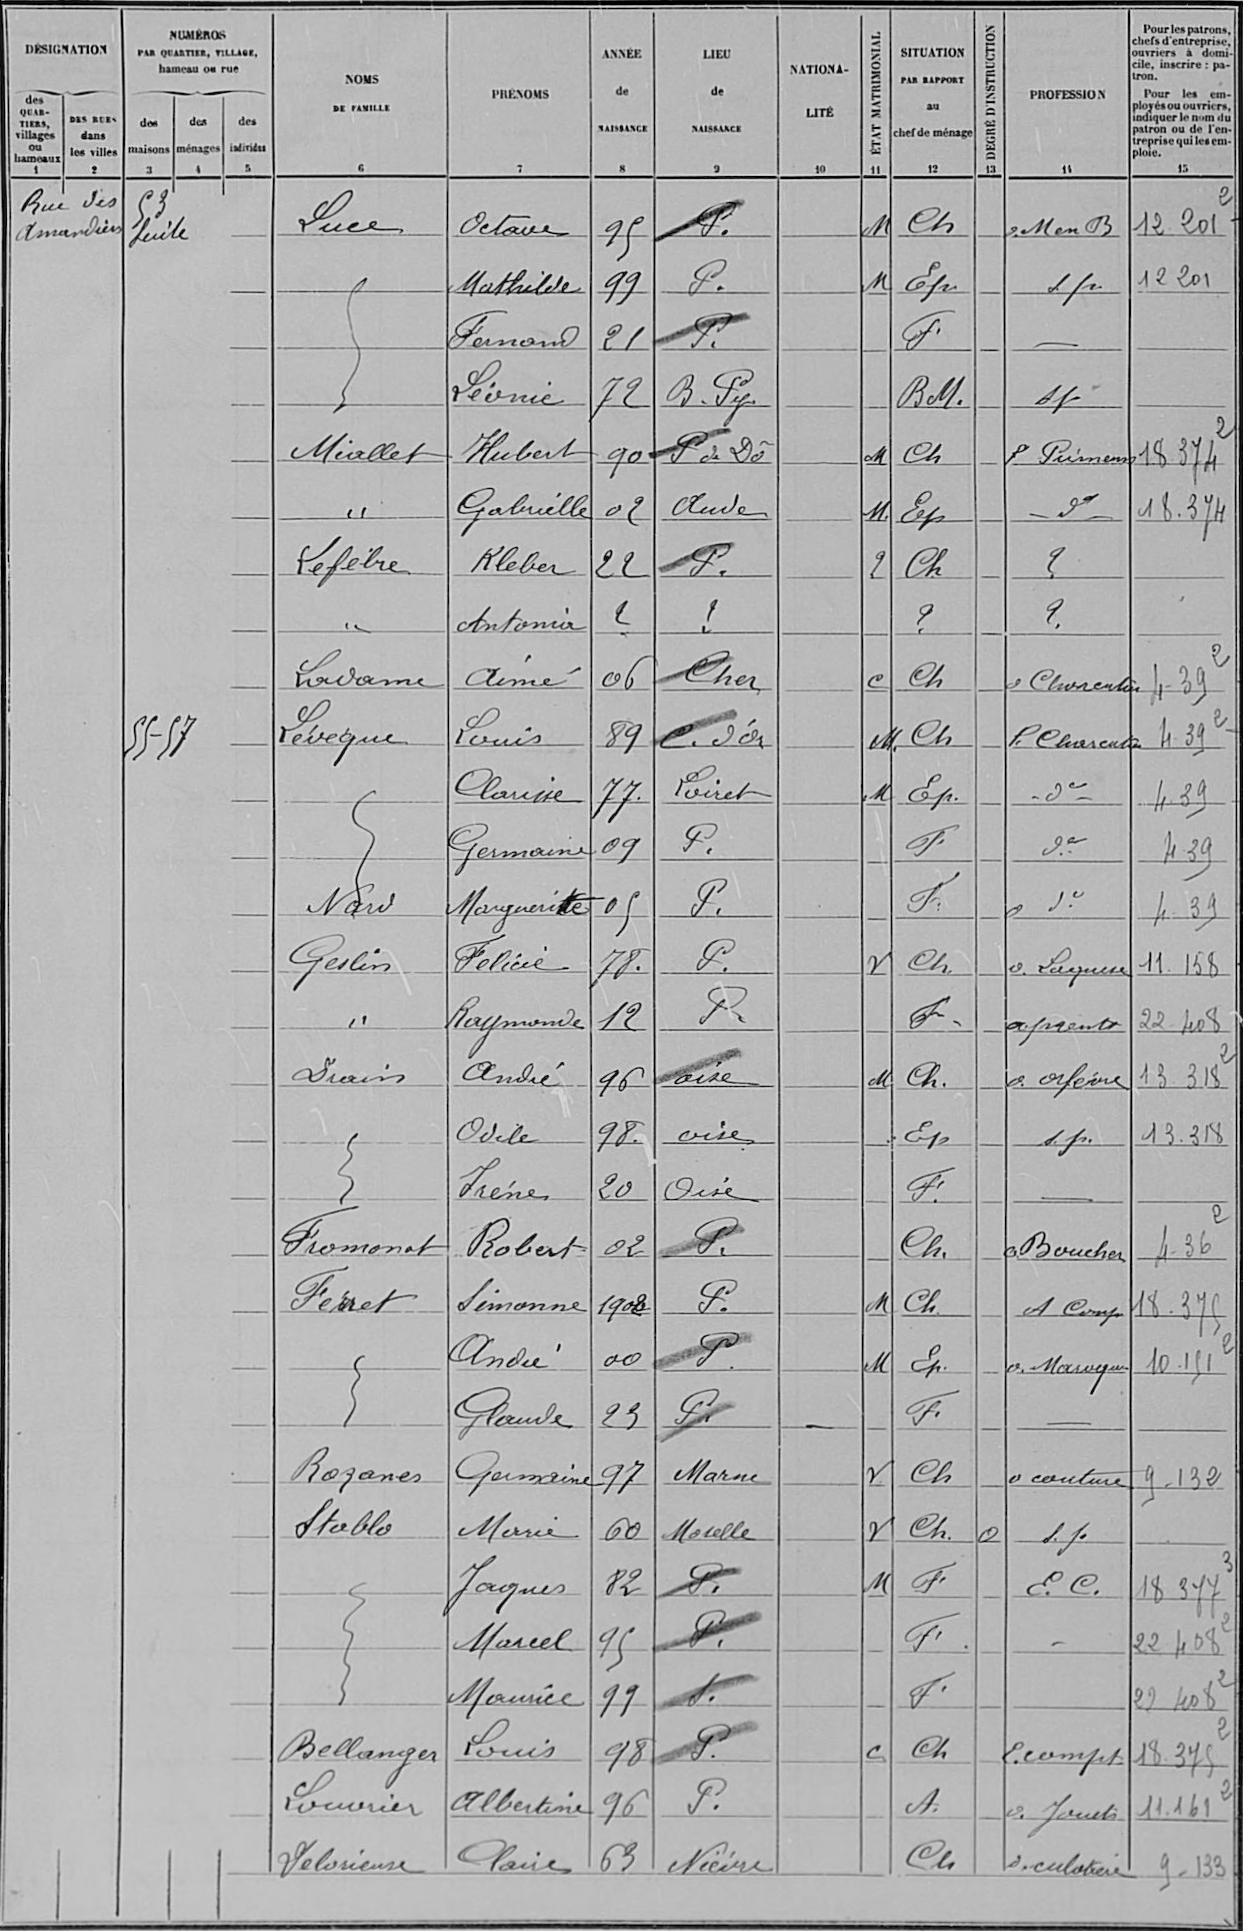Luce/Octave/95/P /¤/M/Ch/¤/Men B/12201
¤/Mathilde/99/P /¤/M/Ep/¤/s p/12201
¤/Fernand/21/P /¤/¤/F/¤/¤/¤
¤/Léonie/72/B Py /¤/¤/BM /¤/sp/¤
Miallet/Hubert/90/P de Do/¤/M/Ch/¤/P Primeurs/18374
''/Gabrielle/02/Aude/¤/M/Ep /¤/d°/18374
Lefebre/Kleber/22/P /¤/¤/Ch/¤/¤/¤
''/Antonin/¤/¤/¤/¤/¤/¤/¤/¤
Louvame/Aimé/06/Cher/¤/C/Ch/¤/o Charcutier/4 39
Léveque/Louis/89/C D'or/¤/M/Ch/¤/P Charcutier/4 39
¤/Clarisse/77/Loiret/¤/M/Ep/¤/d°/4 39
¤/Germaine/09/P /¤/¤/F/¤/d°/439
Naril/Marguerite/05/P /¤/¤/F/¤/d°/4 39
Geslin/Felicie/78/P /¤/V/Ch/¤/o Laqueuse/11158
''/Raymonde/12/P /¤/¤/F/¤/aprenti/22408
Drain/André/96/oise/¤/M/Ch /¤/o orfevre/13318
¤/Odile/98/oise/¤/¤/Ep/¤/s p /13318
¤/Irène/20/Oise/¤/¤/F/¤/¤/¤
Fromonat/Robert/02/P /¤/¤/Ch/¤/o Boucher/436
Fenet/Simonne/1908/P /¤/M/Ch/¤/A Comp/18375
¤/André/00/P /¤/M/Ep/¤/o Maroquin/10151
¤/Claude/23/P /¤/¤/F/¤/¤/¤
Roganes/Germaine/97/Marne/¤/V/Ch/¤/o couture/9132
Stablo/Marie/60/Moselle/¤/V/Ch/O/s p /¤
¤/Jacques/82/P /¤/M/F /¤/E C /18377
¤/Marcel/95/P /¤/¤/F/¤/¤/22408
¤/Maurice/99/P /¤/¤/F/¤/¤/22408
Bellanger/Louis/98/P /¤/c/Ch/¤/E compt/18375
Louvrier/Albertine/96/P /¤/¤/A /¤/o Jouets/11161
Velosienne/Claire/63/Nièvre/¤/¤/Ch/¤/o culotière/9 133
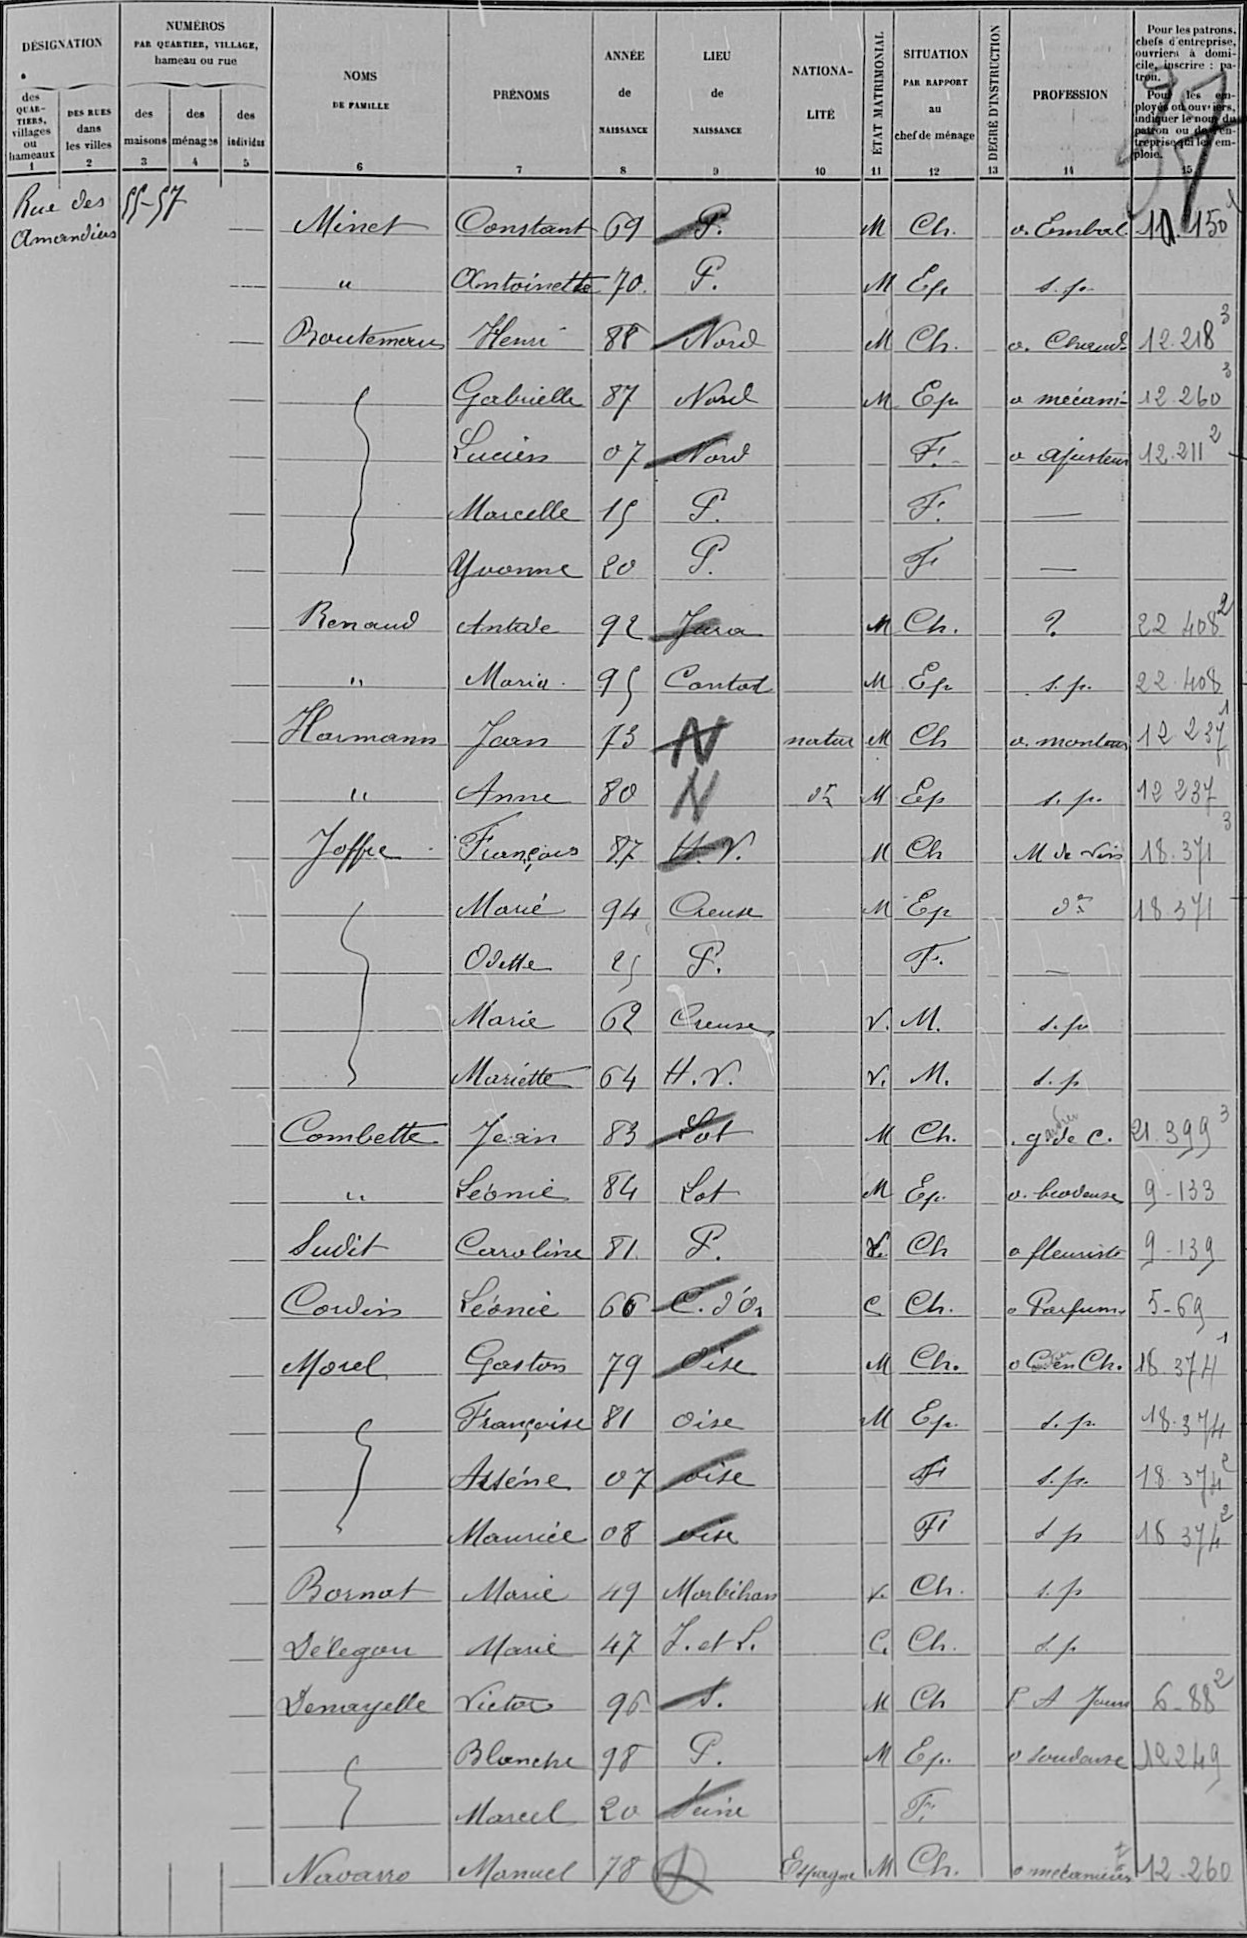Minet/Constant/69/P /¤/M/Ch /¤/o Embal/10 150
¤/Antoinette/70/P /¤/M/Ep/¤/s p /¤
Boutemeris/Henri/88/Nord/¤/M/Ch /¤/o Chaud/12 218
¤/Gabrielle/87/Nord/¤/M/Ep/¤/o mécani/12 260
¤/Lucien/07/Nord/¤/¤/F /¤/o ajusteur/12 211
¤/Marcelle/15/P /¤/¤/F /¤/¤/¤
¤/Yvonne/20/P /¤/¤/F/¤/¤/¤
Renaud/Antale/92/Jura/¤/M/Ch /¤/¤/22408
¤/Maria/95/Cantal/¤/M/Ep/¤/s p /22408
Harmann/Jean/73/¤/natur/M/Ch/¤/a monteur/12237
¤/Anne/80/¤/d°/M/Ep/¤/s p /12 237
Joffre/François/87/H V /¤/M/Ch/¤/M de vins/18 371
¤/Marie/94/Creuse/¤/M/Ep/¤/d°/18 371
¤/Odette/25/P /¤/¤/F /¤/¤/¤
¤/Marie/62/Creuse/¤/V /M /¤/s p /¤
¤/Mariette/64/H V /¤/V /M /¤/s p /¤
Combette/Jean/83/Lot/¤/M/Ch /¤/g de C /21 399
¤/Léonie/84/Lot/¤/M/Ep /¤/o brodeuse/9 133
Suvit/Caroline/81/P /¤/V /Ch/¤/o fleuriste/9 139
Coulin/Léonie/66/C d'O/¤/C/Ch /¤/o Parfums/5 69
Morel/Gaston/79/Oise/¤/M/Ch /¤/o C en Ch /18 374
¤/Françoise/81/Oise/¤/M/Ep /¤/s pr /18 374
¤/Arsène/07/oise/¤/¤/F/¤/s pr /18374
¤/Maurice/08/oise/¤/¤/F/¤/s p/18374
Bornat/Marie/49/Morbihan/¤/V /Ch /¤/s p/¤
Delegau/Marie/47/I et L /¤/C/Ch /¤/s p/¤
Denayelle/Victor/96/S /¤/M/Ch/¤/P A Jours/688
¤/Blanche/98/P /¤/M/Ep /¤/o soudeuse/12249
¤/Marcel/20/Seine/¤/¤/F /¤/¤/¤
Navarro/Manuel/78/¤/Espagne/M/Ch /¤/o mecanicien/12 260